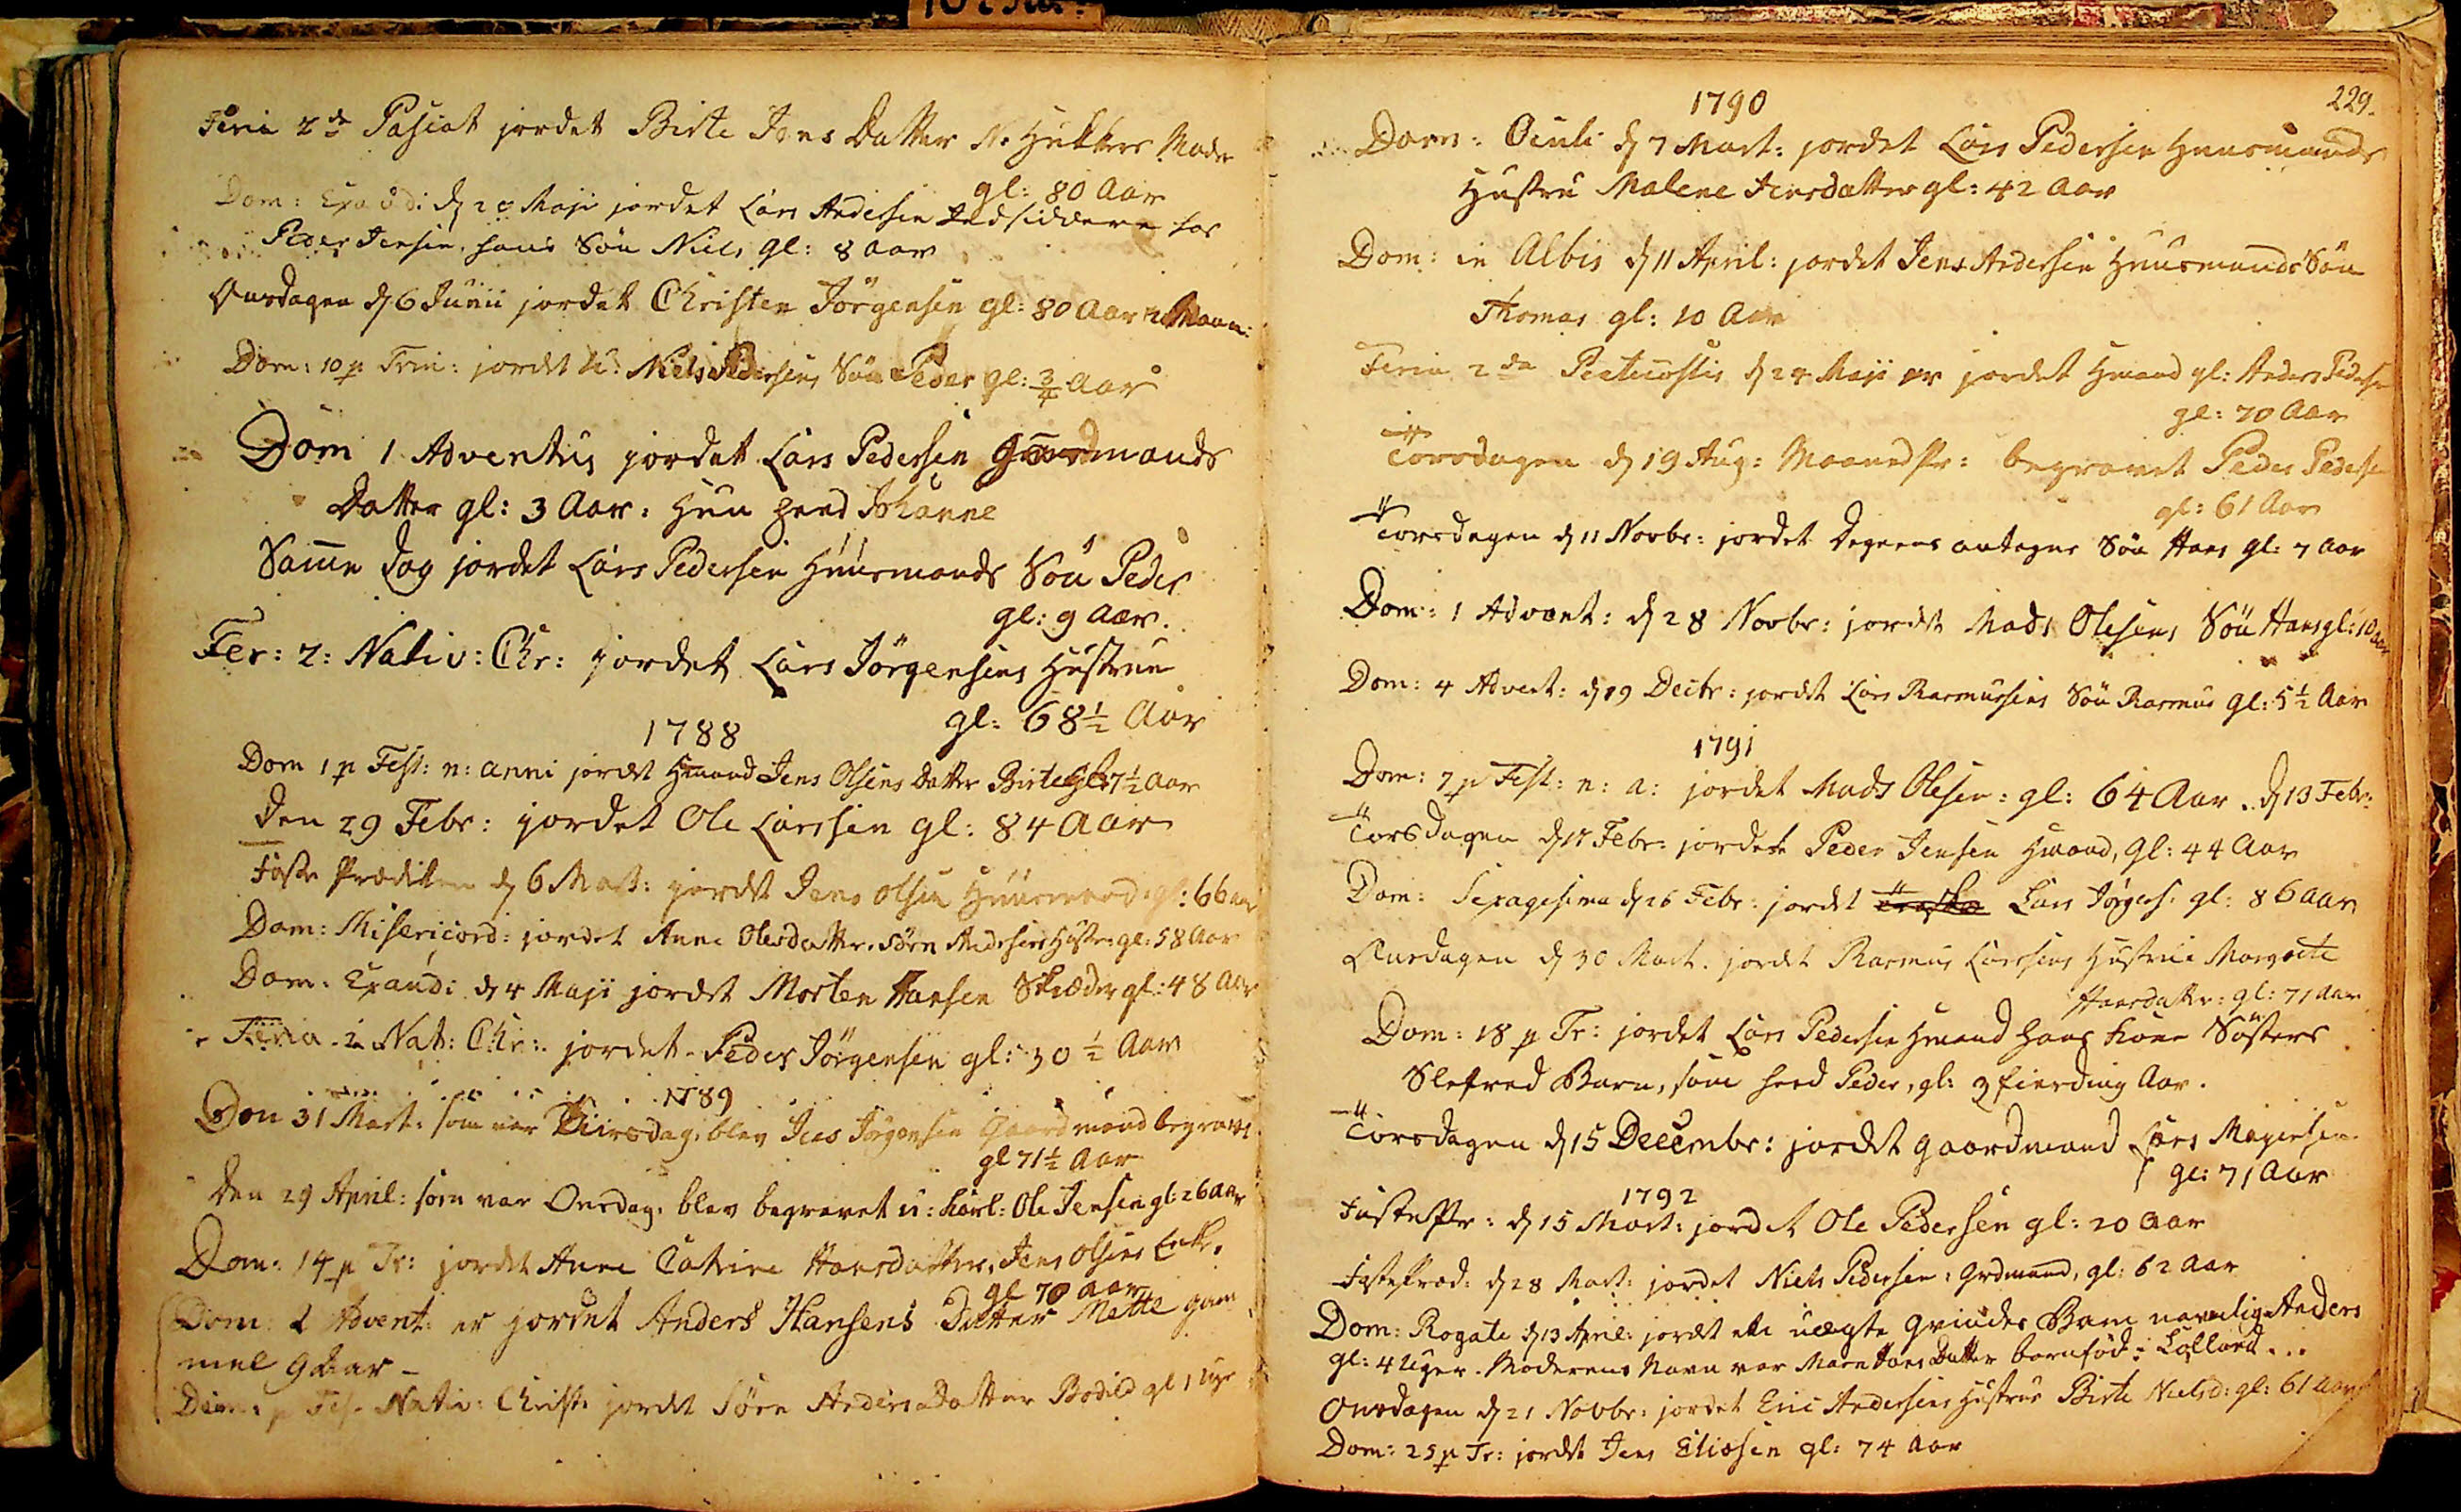Feria 2da Pascat jordet Birte Jens Datter N: Hukkers Moder     
gl: 80 Aar
Dom: Exaudi: d 20 Maji jordet Lars Andersen Indsiddere hos
Peder Jensen, hans Søn Niels gl: 8 Aar
Onsdagen d 6 Junii jordet Christen Jørgensen gl: 80 Aar 2 Maan:
Dom: 10 p Trin: jordet U. Niels Pedersens Søn Peder gl: ¾ Aar
Dom 1 Adventus jordet Lars Pedersen Gaardmands
Datter gl: 3 Aar: Hun heed Johanne
Samme Dag jordet Lars Pedersen Huusmands Søn Peder
gl: 9 Aar.
Fer: 2: Nativ: Chr: jordet Lars Jørgensens Hustrue        
gl: 68½ Aar
1788
Dom 1 p Fest: n: Anni jordet Hmand Jens Olsens Datter Birte Gl 7½ Aar
Den 29 Febr: jordet Ole Larssen gl: 84 Aar
Faste Prædiken d 6 Mart: jordt Jens Olsen Huusmand: gl: 66 Aar
Dom: Misericord: jordet Anne Olesdatter. Sørn Andersens Hustr:   gl: 58 Aar
Dom: Exaudi d 4 Maji jordet Morten Hansen Skræder gl: 48 Aar
Feria. 2 Nat: Chr: jordet Peder Jørgensen gl: 30½ Aar
1789
Den 31 Mart: som var Tiirsdag, blev Jens Jørgensen Gaardmand begravet
gl 71½ Aar
Den 29 April: som var Onsdag: blev begravet u: karl: Ole Jensen gl: 26 Aar
Dom: 14 p Tr: jordet Anne Catrine Hansdatter. Jens Olsens Enke.   
gl 70 Aar
Dom: 2 Advent: er jordet Anders Hansens Datter Mette gam
mel 9 Aar 
Dom: p Fest. Nativ: Christi jordet Sørn Anders Datter Bodild gl 1 Uge
229.
1790
Dom: Oculi d 7 Mart: jordet Lars Pedersen Huusmands
Hustru Malene Jensdatter                    gl: 42 Aar
Dom: in Albis d 11 April: jordet Jens Andersen Huusmands Søn
Thomas gl: 10 Aar
Feria 2da Pentecostis d 24 maji er jordet Hmand gl: Anders Pedersen
gl: 70 Aar
Torsdagen d 19 Aug: Maaned Pr: begravet Peder Pedersen       
gl: 61 Aar
Torsdagen d 11 Novbr: jordet Degnens antagne Søn Hans gl: 7 Aar
Dom: 1 Advent: d 28 Novbr: jordet Mads Olesens Søn Hans gl: 10 Aar
Dom: 4 Advent: d 19 Decbr: jordet Lars Rasmussens Søn Rasmus gl: 5½ Aar
1791
Dom: 7 p Fest: n: a: jordet Mads Olesen: gl: 64 Aar. d 13 Febr:
Torsdagen d 17 Febr: jordet Peder Jensen Hmand, gl: 44 Aar
Dom: Sexagesima d 26 Febr: jordet Træsko Lars Jørgens: gl: 86 Aar
Onsdagen d 30 Mart: jordet Rasmus Larssens Hustrue Margrete
Hansdatter: gl: 71 Aar
Dom: 18 p Tr: jordet Lars Pedersen Hmand hans Kone Søsters
Slefred Barn, som heed Peder, gl: 3 fierding Aar.
Torsdagen d 15 Decembr: jordt Gaardmand Lars Mogensen
gl: 71 Aar
1792
Fastepr: d 15 Mart: jordet Ole Pedersen gl: 20 Aar
Fastepræd: d 28 Mart: jordet Niels Pedersen: Grdmand, gl: 62 Aar
Dom: Rogate d 13 April: jordt en uægte Qvindes Barn navnlig Anders
gl: 4 Uger. Moderens Navn var Marn Hans Datter barnefød i Lolland. . .
Onsdagen d 21 Novbr: jordet Eric Andersens Hustrue Birte Nielsd:  gl: 61 Aar
Dom: 25 p Tr: jordt Jens Eliæsen gl: 74 Aar
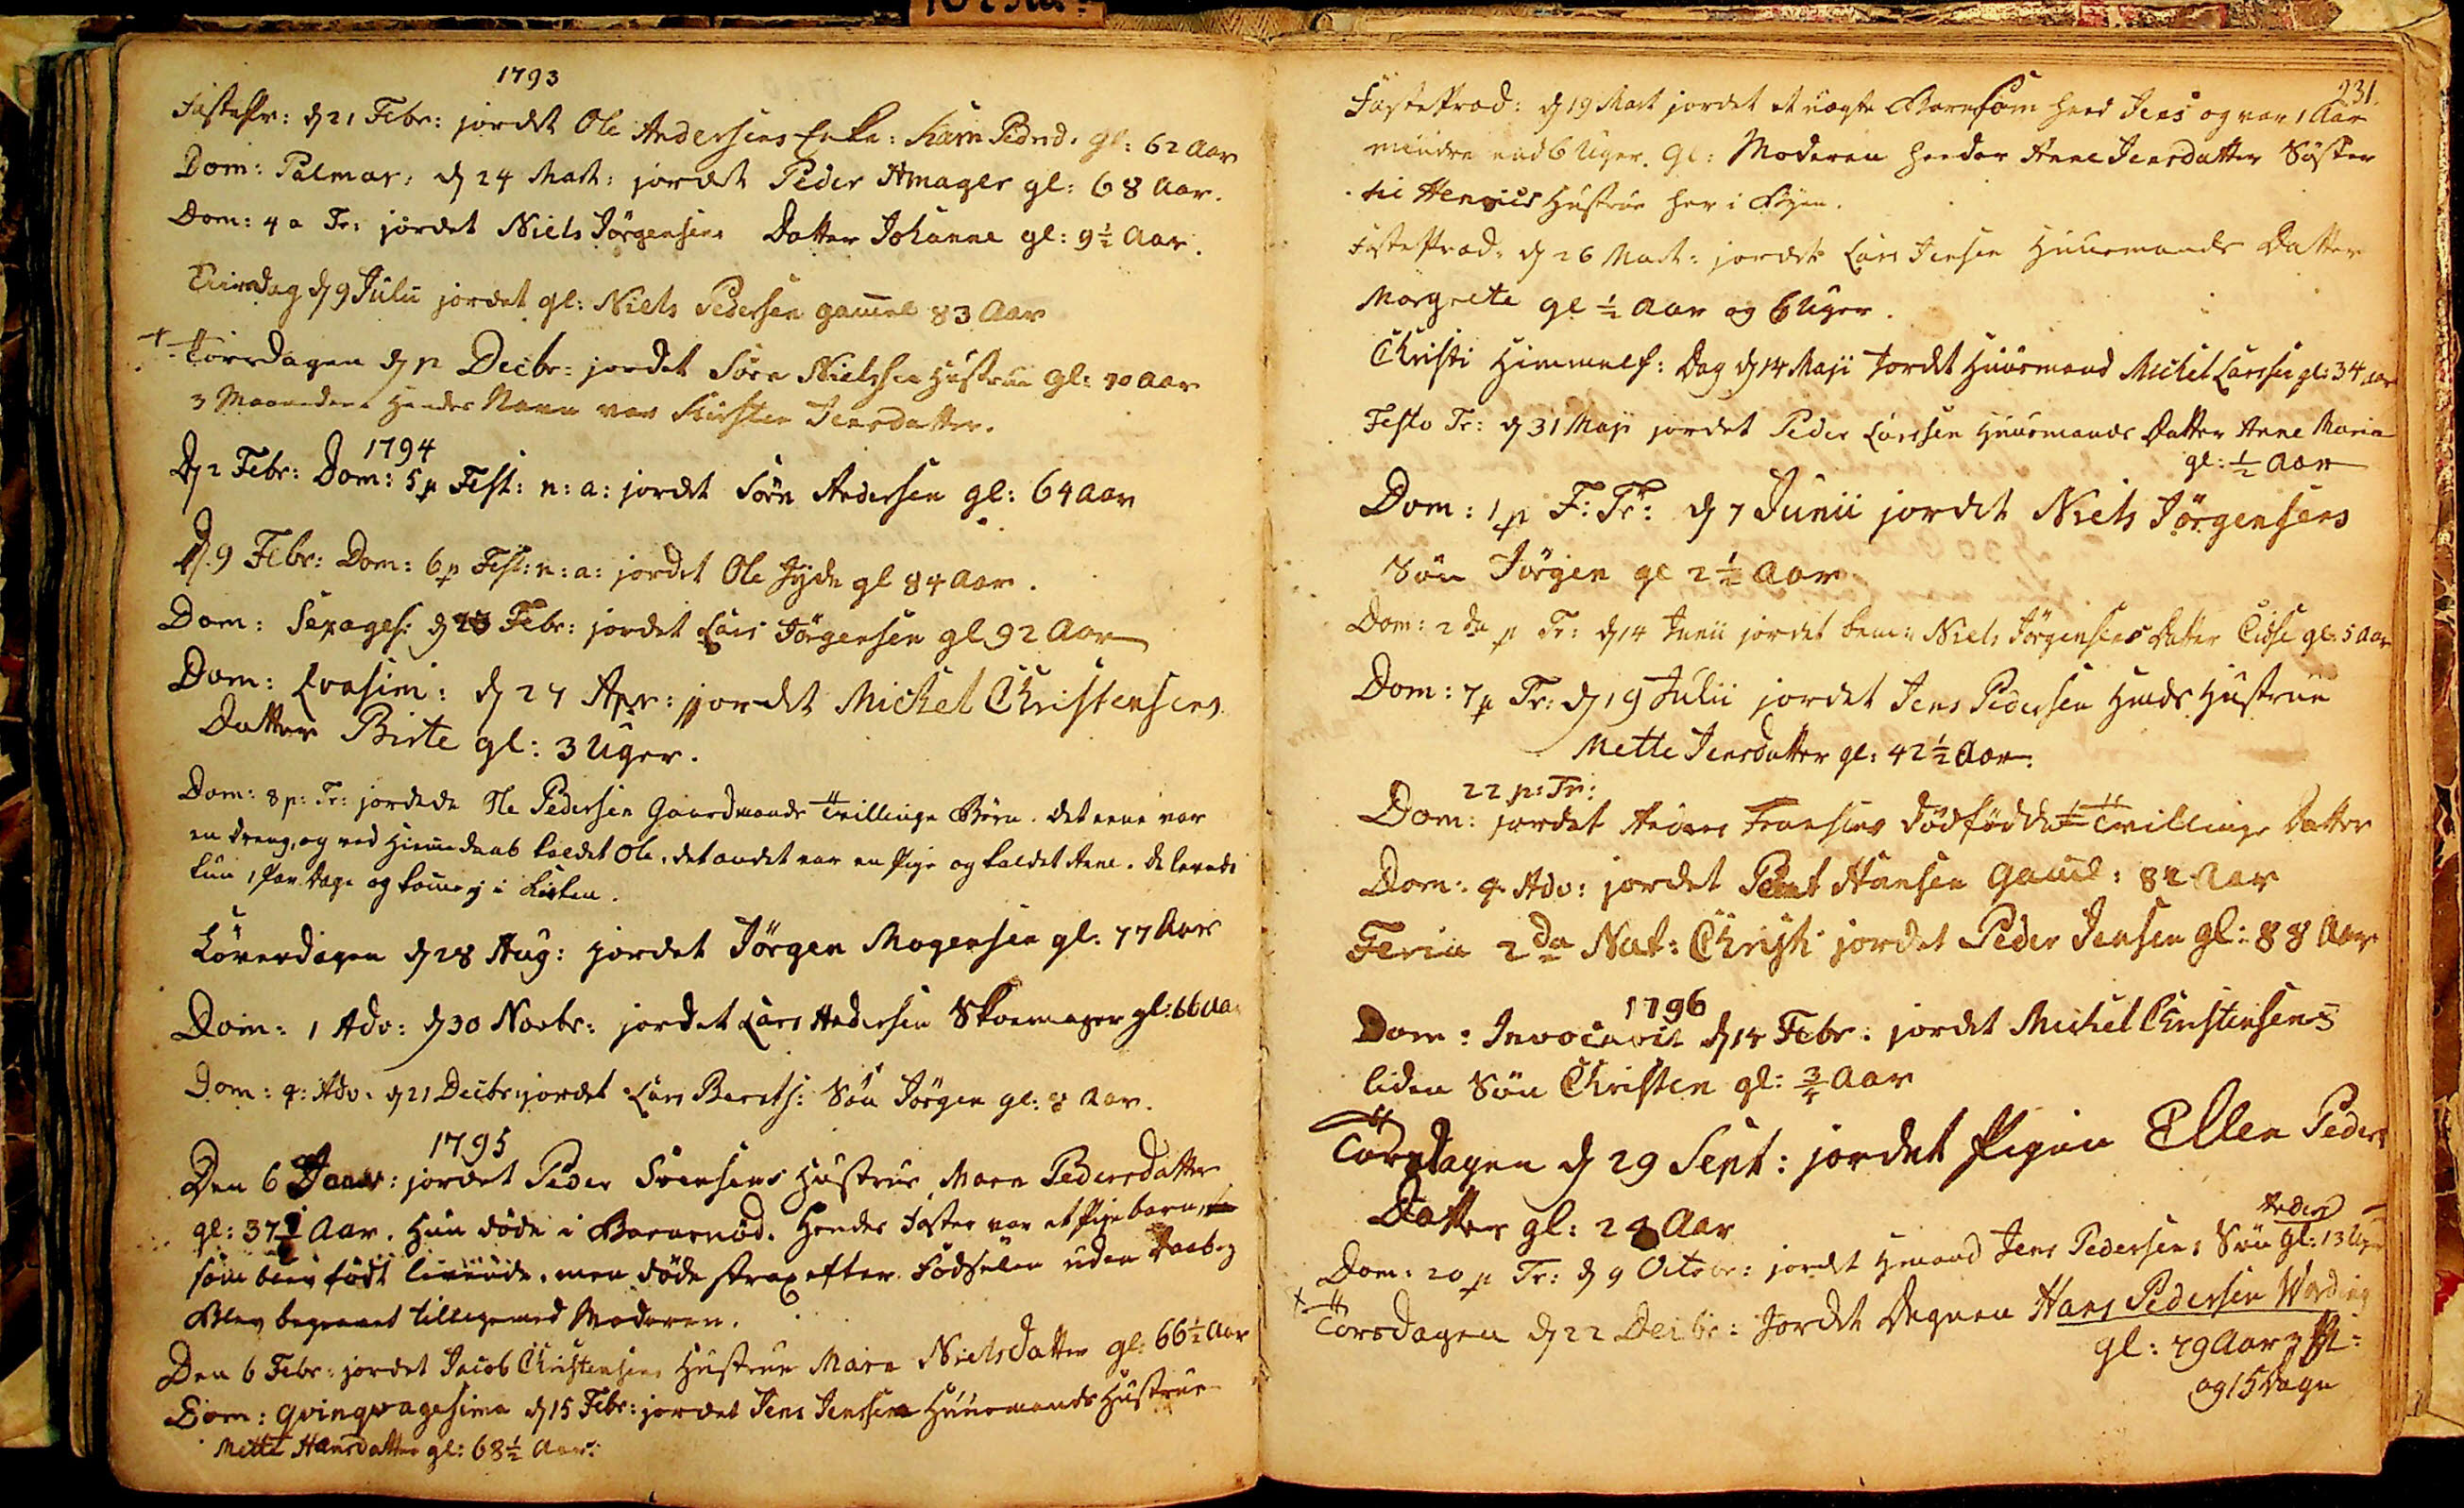1793
Fastepr: d 21 Febr: jordt Ole Andersens Enke: Karn Pedersd: gl: 62 Aar
Dom: Palmar: d 24 Mart: jordet Peder Amager gl: 68 Aar.
Dom 4 a Tr: jordet Niels Jørgensens Datter Johanne gl: 9½ Aar
Tiirsdag d 9 Julii jordet gl: Niels Pedersen gammel 83 Aar
Torsdagen d 12 Decbr: jordet Sørn Nielssen Hustrue gl: 40 Aar
3 Maaneder. Hendes Navn var Kirsten Jensdatter.
1794
d 2 Febr: Dom: 5 p Fest: n: a: jordt Sørn Andersen gl: 64 Aar
d. 9 Febr: Dom: 6 p Fest: n: a: jordet Ole Jyde gl 84 Aar.
Dom: Sexages: d 23 Febr: jordet Lars Jørgensen gl 92 Aar
Dom: Qvasim: d 27 Apr: jordet Michel Christensens 
Datter Birte  gl: 3 Uger.
Dom: 8 p : Tr: jordede Ole Pedersen Gaardmands Tvillinge Børn. Det eene var
en Dreng, og ved Hiemmedaab kaldet Ole, det andet var en Pige og kaldet Anne. De
levede kun 1 Par Dage og komme ej i Kirken.
Løverdagen d 28 Aug: jordet Jørgen Mogensen gl. 77 Aar
Dom: 1 Adv: d 30 Novbr: jordet Lars Andersens Skoemager gl: 66 Aar
Dom: 4: Adv: d 21 Decbr: jordet Lars Bernts: Søn Jørgen gl: 8 Aar.
1795
Den 6 Janu: jordet Peder Svensens Hustrue Marn Pedersdattter
gl: 37½ Aar. Hun døde i Barnenød. Hendes Foster var et Pigebarn
som blev født levende, men døde strax efter Fødselen uden Daab og
Blev begravet tilligemed Moderen.
Den 6 Febr: jordet Jacob Christensens Hustrue Marn Nielsdatter  gl: 66½ Aar
Dom: Qvinqvagesima d 15 Febr: jordet Jens Jenssen Huusmands Hustrue
Mette Hansdatter gl: 68½ Aar:
231.
Fastepræd: d 19 Mart jordet et uægte Barn som heed Jens og var 1 Aar
mindre end 6 Uger Gl: Moderen heeder Anne Jensdatter Søster
til Henrics hustrue her i Byen.
Fastepræd: d 26 Mart: jordet Lars Jensen Huusmands Datter
Margrete gl ½ Aar og 6 Uger.
Christi Himmelf: Dag d 14 Maji jordt Huusmand Michel Larssen gl: 34 Aar
Festo Tr: d 31 Maji jordet Peder Larssen Huusmands Datter Anne Marie
gl: ½ Aar
Dom: 1 p F: Tr: d 7 Junii jordet Niels Jørgensens 
søn Jørgen gl 2½ Aar
Dom: 2da p Tr: d 14 Junii jordet bem: Niels Jørgensens Datter Cidse gl: 5 Aar
Dom: 7 p Tr: d 19 Julii jordet Jens Pedersen Hmds Hustrue
Mette Jensdatte gl: 42½ Aar
22 p: Tr:
Dom: jordet Anders Fransens dødfødde Tvillinge Datter
Dom: 4 Adv: jordet Beent Hansen Gammel: 82 Aar
Feria 2da Nat: Christi jordet Peder Jensen gl: 88 Aar
1796
Dom: Invocavit d 14 Febr: jordet Michel Christensens
liden Søn Christen gl: ¾ Aar
Torsdagen d 29 Sept: jordet Pigen Ellen Peders 
Datter gl: 24 Aar
Anders
Dom: 20 p Tr: d 9 Octobr: jordet Hmand Jens Pedersens Søn gl: 13 Uger
Torsdagen d 22 Decbr: jordt Degnen Hans Pedersen Wording
gl: 79 Aar 3 M: 
og 15 Dage